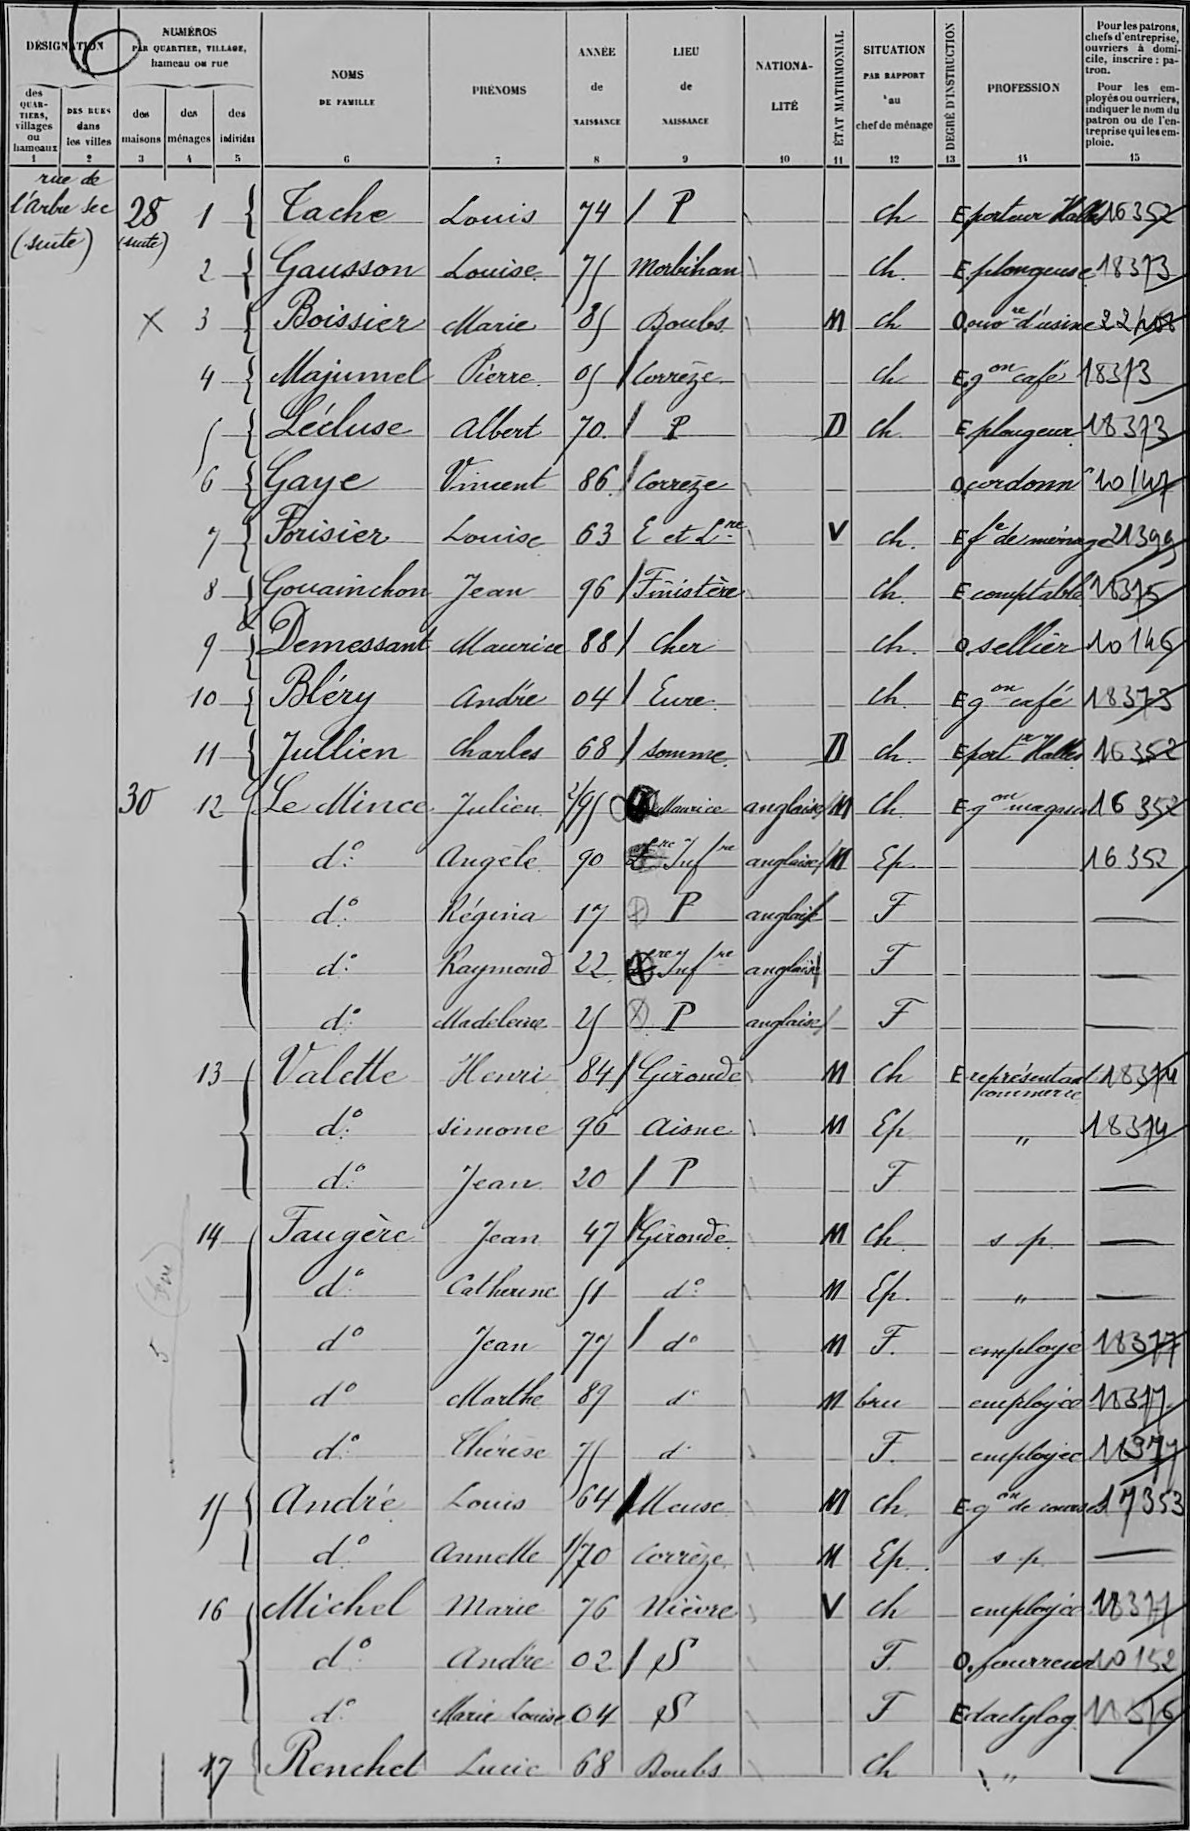Tache/Louis/74/P/¤/¤/ch/¤/E porteur Halles/16352
Gausson/Louise/75/Morbihan/¤/¤/ch/¤/E plongeuse/18373
Boissier/Marie/85/Doubs/¤/M/ch/¤/O ouv d'usine/22408
Majumel/Pierre/05/Corrèze/¤/¤/ch/¤/E gon café/18373
Lécluse/Albert/70/P/¤/D/Ch/¤/E plongeur/18373
Gaye/Vincent/86/Corrèze/¤/¤/¤/¤/o cordonn/10147
Forisier/Louise/63/E et Lre/¤/V/Ch/¤/E f de ménage/21399
Gouainchon/Jean/96/Finistère/¤/¤/Ch/¤/E comptable/18375
Demessant/Maurice/88/Cher/¤/¤/Ch/¤/o sellier/10146
Bléry/André/04/Eure/¤/¤/Ch /¤/E gon café/18373
Jullien/Charles/68/Somme/¤/D/Ch/¤/E port Halles/16352
Le Mince/Julien/2:95/Ile Maurice/anglaise/M/Ch/¤/E gon magasin/16352
d°/Angèle/90/Lre Infre/anglaise/M/Ep/¤/¤/16352
d°/Régina/17/P/anglaise/¤/F/¤/¤/¤
d°/Raymond/22/Lre Infre/anglaise/¤/F/¤/¤/¤
d°/Madeleine/25/P/anglaise/¤/F/¤/¤/¤
Valette/Henri/84/Gironde/¤/M/Ch/¤/E représentant !commerce/18374
d°/Simone/96/Aisne/¤/M/Ep/¤/''/18374
d°/Jean/20/P/¤/¤/F/¤/¤/¤
Faugère/Jean/47/Gironde/¤/M/Ch/¤/sp/¤
d°/Catherine/51/d°/¤/M/Ep /¤/''/¤
d°/Jean/77/d°/¤/M/F/¤/employé/18377
d°/Marthe/89/d°/¤/M/bru/¤/employée/18377
d°/Thérèse/75/d°/¤/¤/F/¤/employée/11977
André/Louis/64/Meuse/¤/M/ch/¤/E gon de courses/17353
d°/Annette/1:70/Corrèze/¤/M/Ep/¤/s p/¤
Michel/Marie/76/Nièvre/¤/V/ch/¤/employée/18377
d°/André/02/S/¤/¤/F/¤/O fourreur/10152
d°/Marie Louise/04/S/¤/¤/F/¤/E dactylog/18376
Renchet/Lucie/68/Doubs/¤/¤/Ch/¤/''/¤
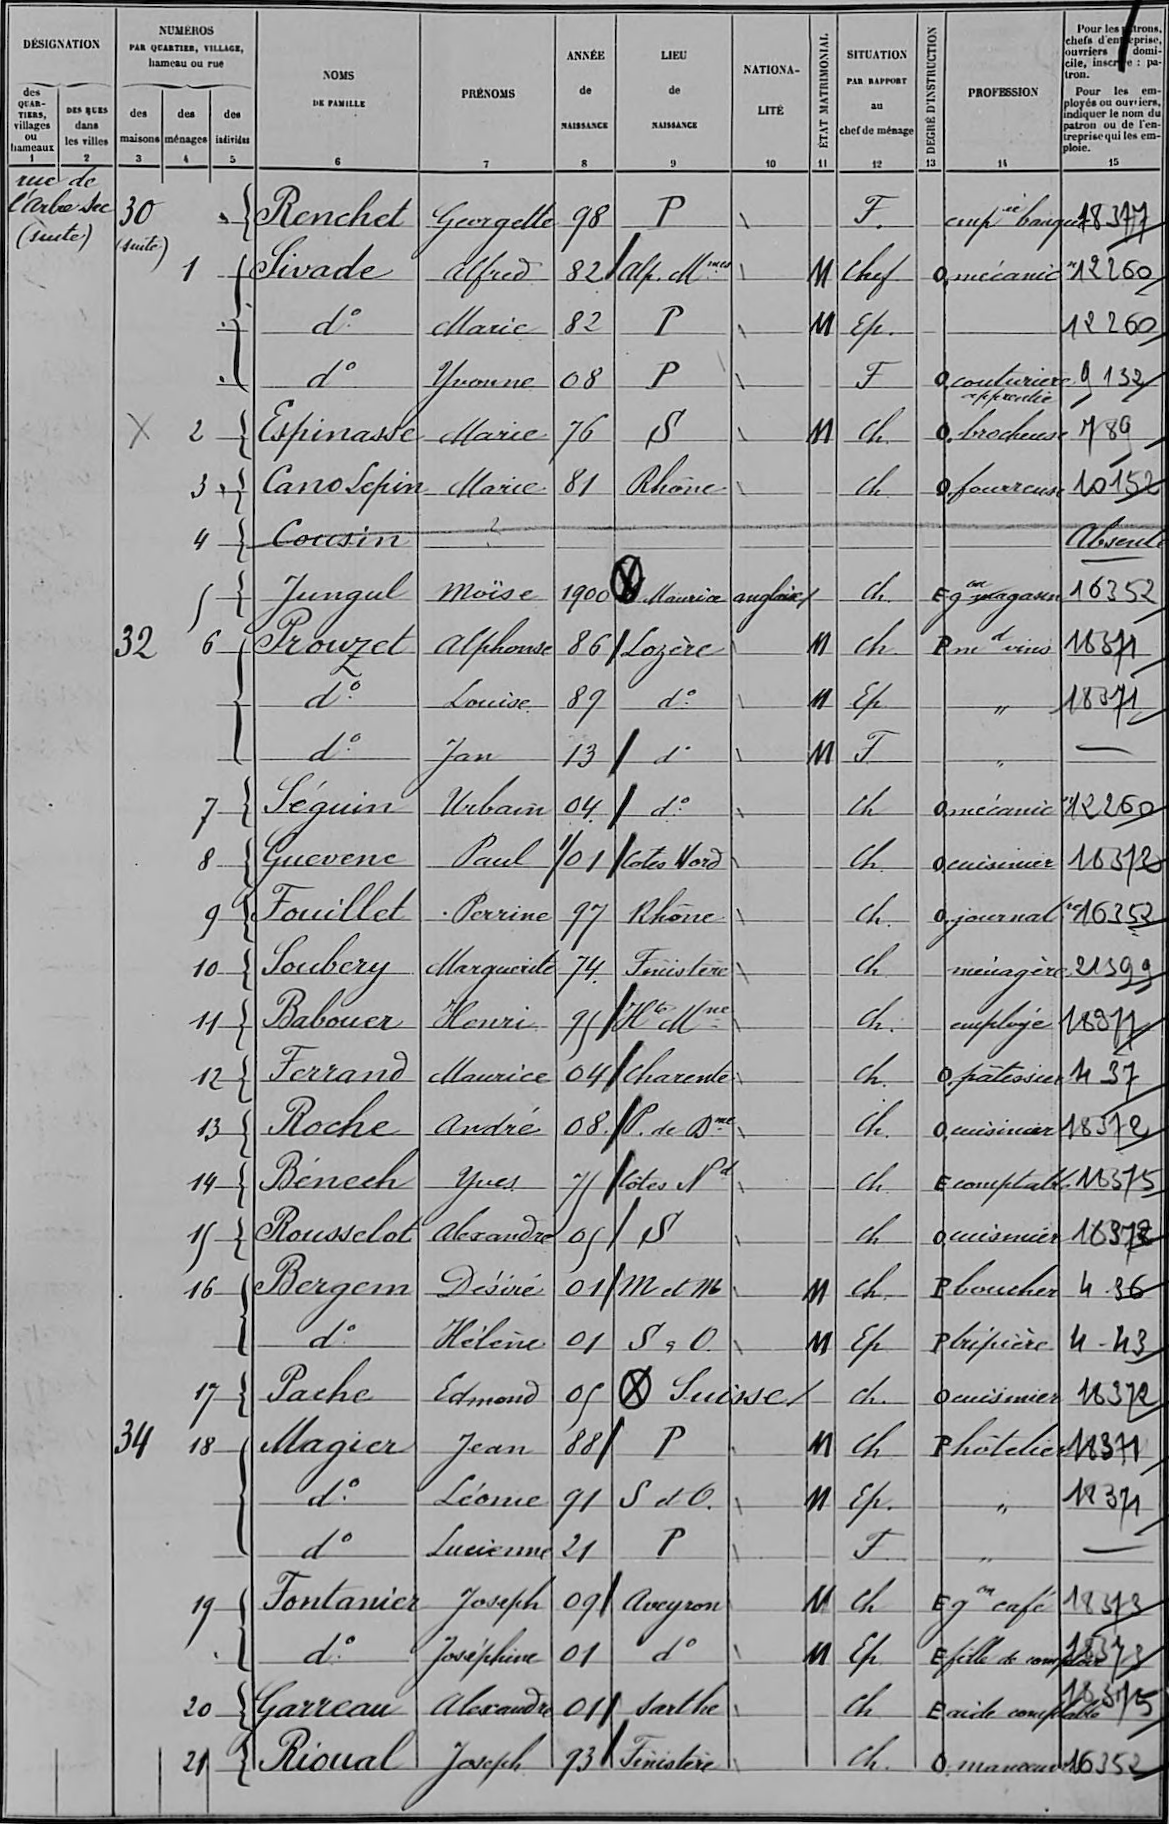Renchet/Georgette/98/P/¤/¤/F /¤/emplée banque/18377
Sivade/Alfred/82/Alp Mmes/¤/M/Chef/¤/O mécanic/12260
d°/Marie/82/P/¤/M/Ep /¤/¤/12260
d°/Yvonne/08/P/¤/¤/F/¤/O couturiere !apprentie/9132
Espinasse/Marie/76/S/¤/M/Ch/¤/O brocheuse/789
Cano Lepin/Marie/81/Rhône/¤/¤/Ch/¤/O fourreuse/10152
Cousin/¤/¤/¤/¤/¤/¤/¤/¤/Absent
Jungul/Moïse/1900/Ile Maurice/anglaise/¤/Ch /¤/E g magasin/16352
Prouzet/Alphonse/86/Lozère/¤/M/Ch/¤/P m d vins/18371
d°/Louise/89/d°/¤/M/Ep/¤/''/18371
d°/Jan/13/d°/¤/M/F/¤/''/¤
Séguin/Urbain/04/d°/¤/¤/Ch/¤/O mécanic/12260
Guevenc/Paul/1:01/Cotes Nord/¤/¤/Ch/¤/o cuisinier/18372
Fouillet/Perrine/97/Rhône/¤/¤/Ch/¤/o journal/16352
Soubery/Marguerite/74/Finistère/¤/¤/Ch/¤/ménagère/21399
Babouer/Henri/95/Hte Mne/¤/¤/Ch/¤/employé/18377
Ferrand/Maurice/04/Charente/¤/¤/Ch/¤/O pâtissier/437
Roche/andré/08/P de Dme/¤/¤/Ch/¤/O cuisinier/18372
Bénech/Yves/75/Côtes Nd/¤/¤/Ch/¤/E comptable/18375
Rousselot/Alexandre/05/S/¤/¤/Ch/¤/o cuisinier/18372
Bergem/Désiré/01/M et M/¤/M/Ch/¤/P boucher/436
d°/Hélène/01/S & O/¤/M/Ep/¤/P tripière/443
Pache/Edmond/05/Suisse/¤/¤/Ch/¤/O cuisinier/18372
Magier/Jean/88/P/¤/M/Ch/¤/P hôtelier/18371
d°/Léonie/91/S et O/¤/M/Ep/¤/''/18371
d°/Lucienne/21/P/¤/¤/F/¤/''/¤
Fontanier/Joseph/09/Aveyron/¤/M/Ch/¤/E gon café/18373
d°/Joséphine/01/d°/¤/M/Ep/¤/E fille de comp/18373
Garreau/Alexandre/01/Sarthe/¤/¤/Ch/¤/E aide comptable/?18375
Rioual/Joseph/93/Finistère/¤/¤/Ch/¤/O manoeuvre/16352